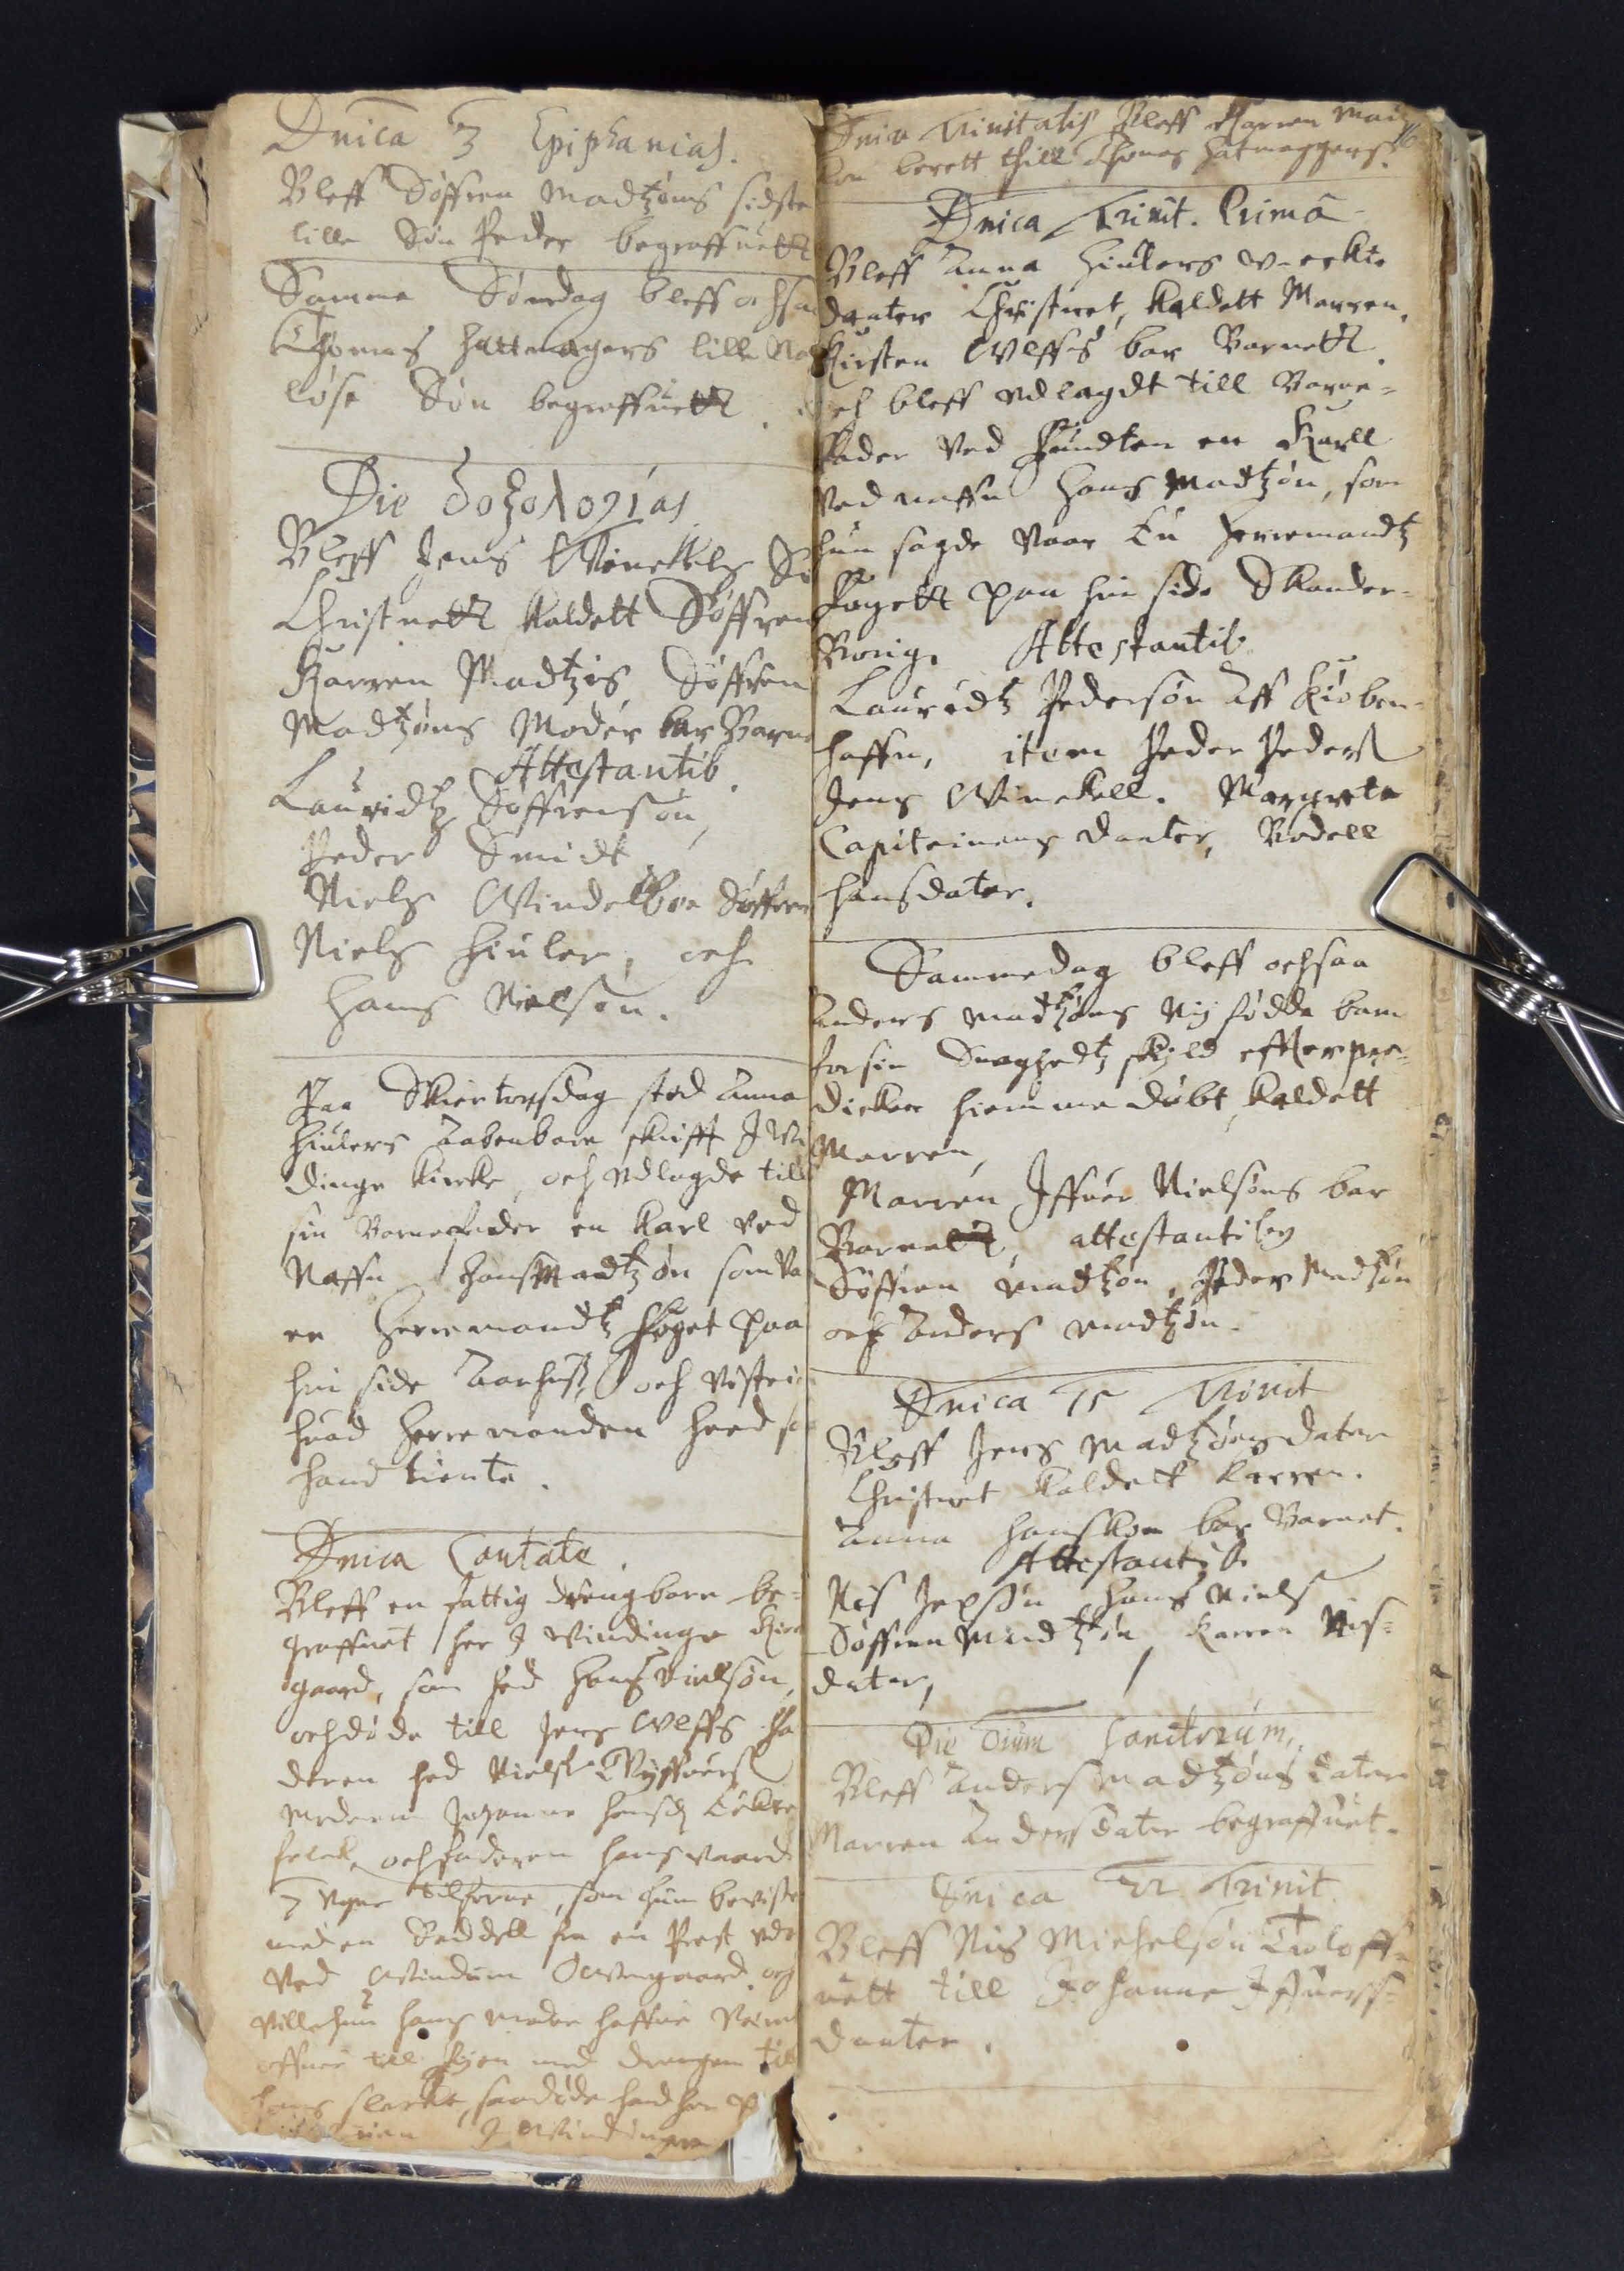Dnica 3 Epiphanias.
Bleff Søffren Madtzøns sidste
lille Søn Peder begraffuett
Samme Søndag bleff ochsaa
Thomas Hattemagers lille na 
løse Søn begraffuett
Die ##
Bleff Jens Winckels S 
Christnett kaldett Søffren
Karren Madtzis Søffren
Madtzøns Moder bar Barnet.
Attestantib
Lauridtz Søffrensøn
Peder Smidt
Niels Windelboe Søffren
Niels Hiuler, och
Hans Nielsøn
Paa Skiertorsdag stod Anna
Hiulers Aabenbare skrift I Wi  
dinge Kircke, och vdlagde till
sin Barnefader en karl ved
Naffn Hans Madtzøn som va¬
en Herremandtz Foget paa
hin side Aarhuss, och viste ic 
huad Herremanden heed
hand tiente
Dnica Cantate
Bleff en fattig drengebarn be¬
graffuet her I Windinge Kirc 
gaard, som hed Hans Nielsøn
och døde till Jens Vlffs. Fa
deren hed Niels Gijffuers
Moderen Johanne Hansd. Eckte
folck och faderen hans vaard
7 Vger tilforne## som hun beviste
med en Seddel fra en Præst
ved ##stind## ##stergaard och
ville hun hans moder haffue varet
pffuer til Fyen med drengen til
hans sleckt, saa døde hand for
## ## ## ##
16
Dnica Trinitatis. Bleff Karren Madtz
Kon berett thill Thomas Hatmaggers.
Dnica Trinit. Prima
Bleff Anna Hiulers v-eckte
daater Christnet, kaldett Marren
Kirsten Vlffs bar Barnett
 ch bleff udlagdt till Barne¬
fader ved Fundten en karll
ved naffn Hans Madtzøn, som
hun sagde vaar En Herremandtz
Fogett paa hin side Skander¬
Borig.
Attestantil
Lauridtz Pedersøn Aff Kiøben¬
haffn, item Peder Peders
Jens Winckell. Margreta
Capitainens daater. Bodell
Hansdaater.
Sammedag bleff ochsaa
Anders Madtzøns Ny fødde barn
for sin Suaghedtz skyld effter
dicken hiemme døbt, kaldett
Marren
Marren Iffuer Nielsøns bar
Barnett, Attestantiby
Søffren Madtzøn. Peder Madtzøn
och Anders Madtzøn
Dnica 15 Trinit
Bleff Jens Madtzøns dater
Christnet kaldett Karren.
Anna Hans Kone bar Barnet
Attestantib.
Nis Jepsøn Hans Niels
Søffren Madtzøn, Karren Nis¬
dater
Die Oium sanctorum.
Bleff Anders Madtzøns dater
Marren Andersdater begraffuet.
Dnica 22 Trinit.
Bleff Nis Michelsøn Troloff¬
uett till Johanne Iffuers¬
daater
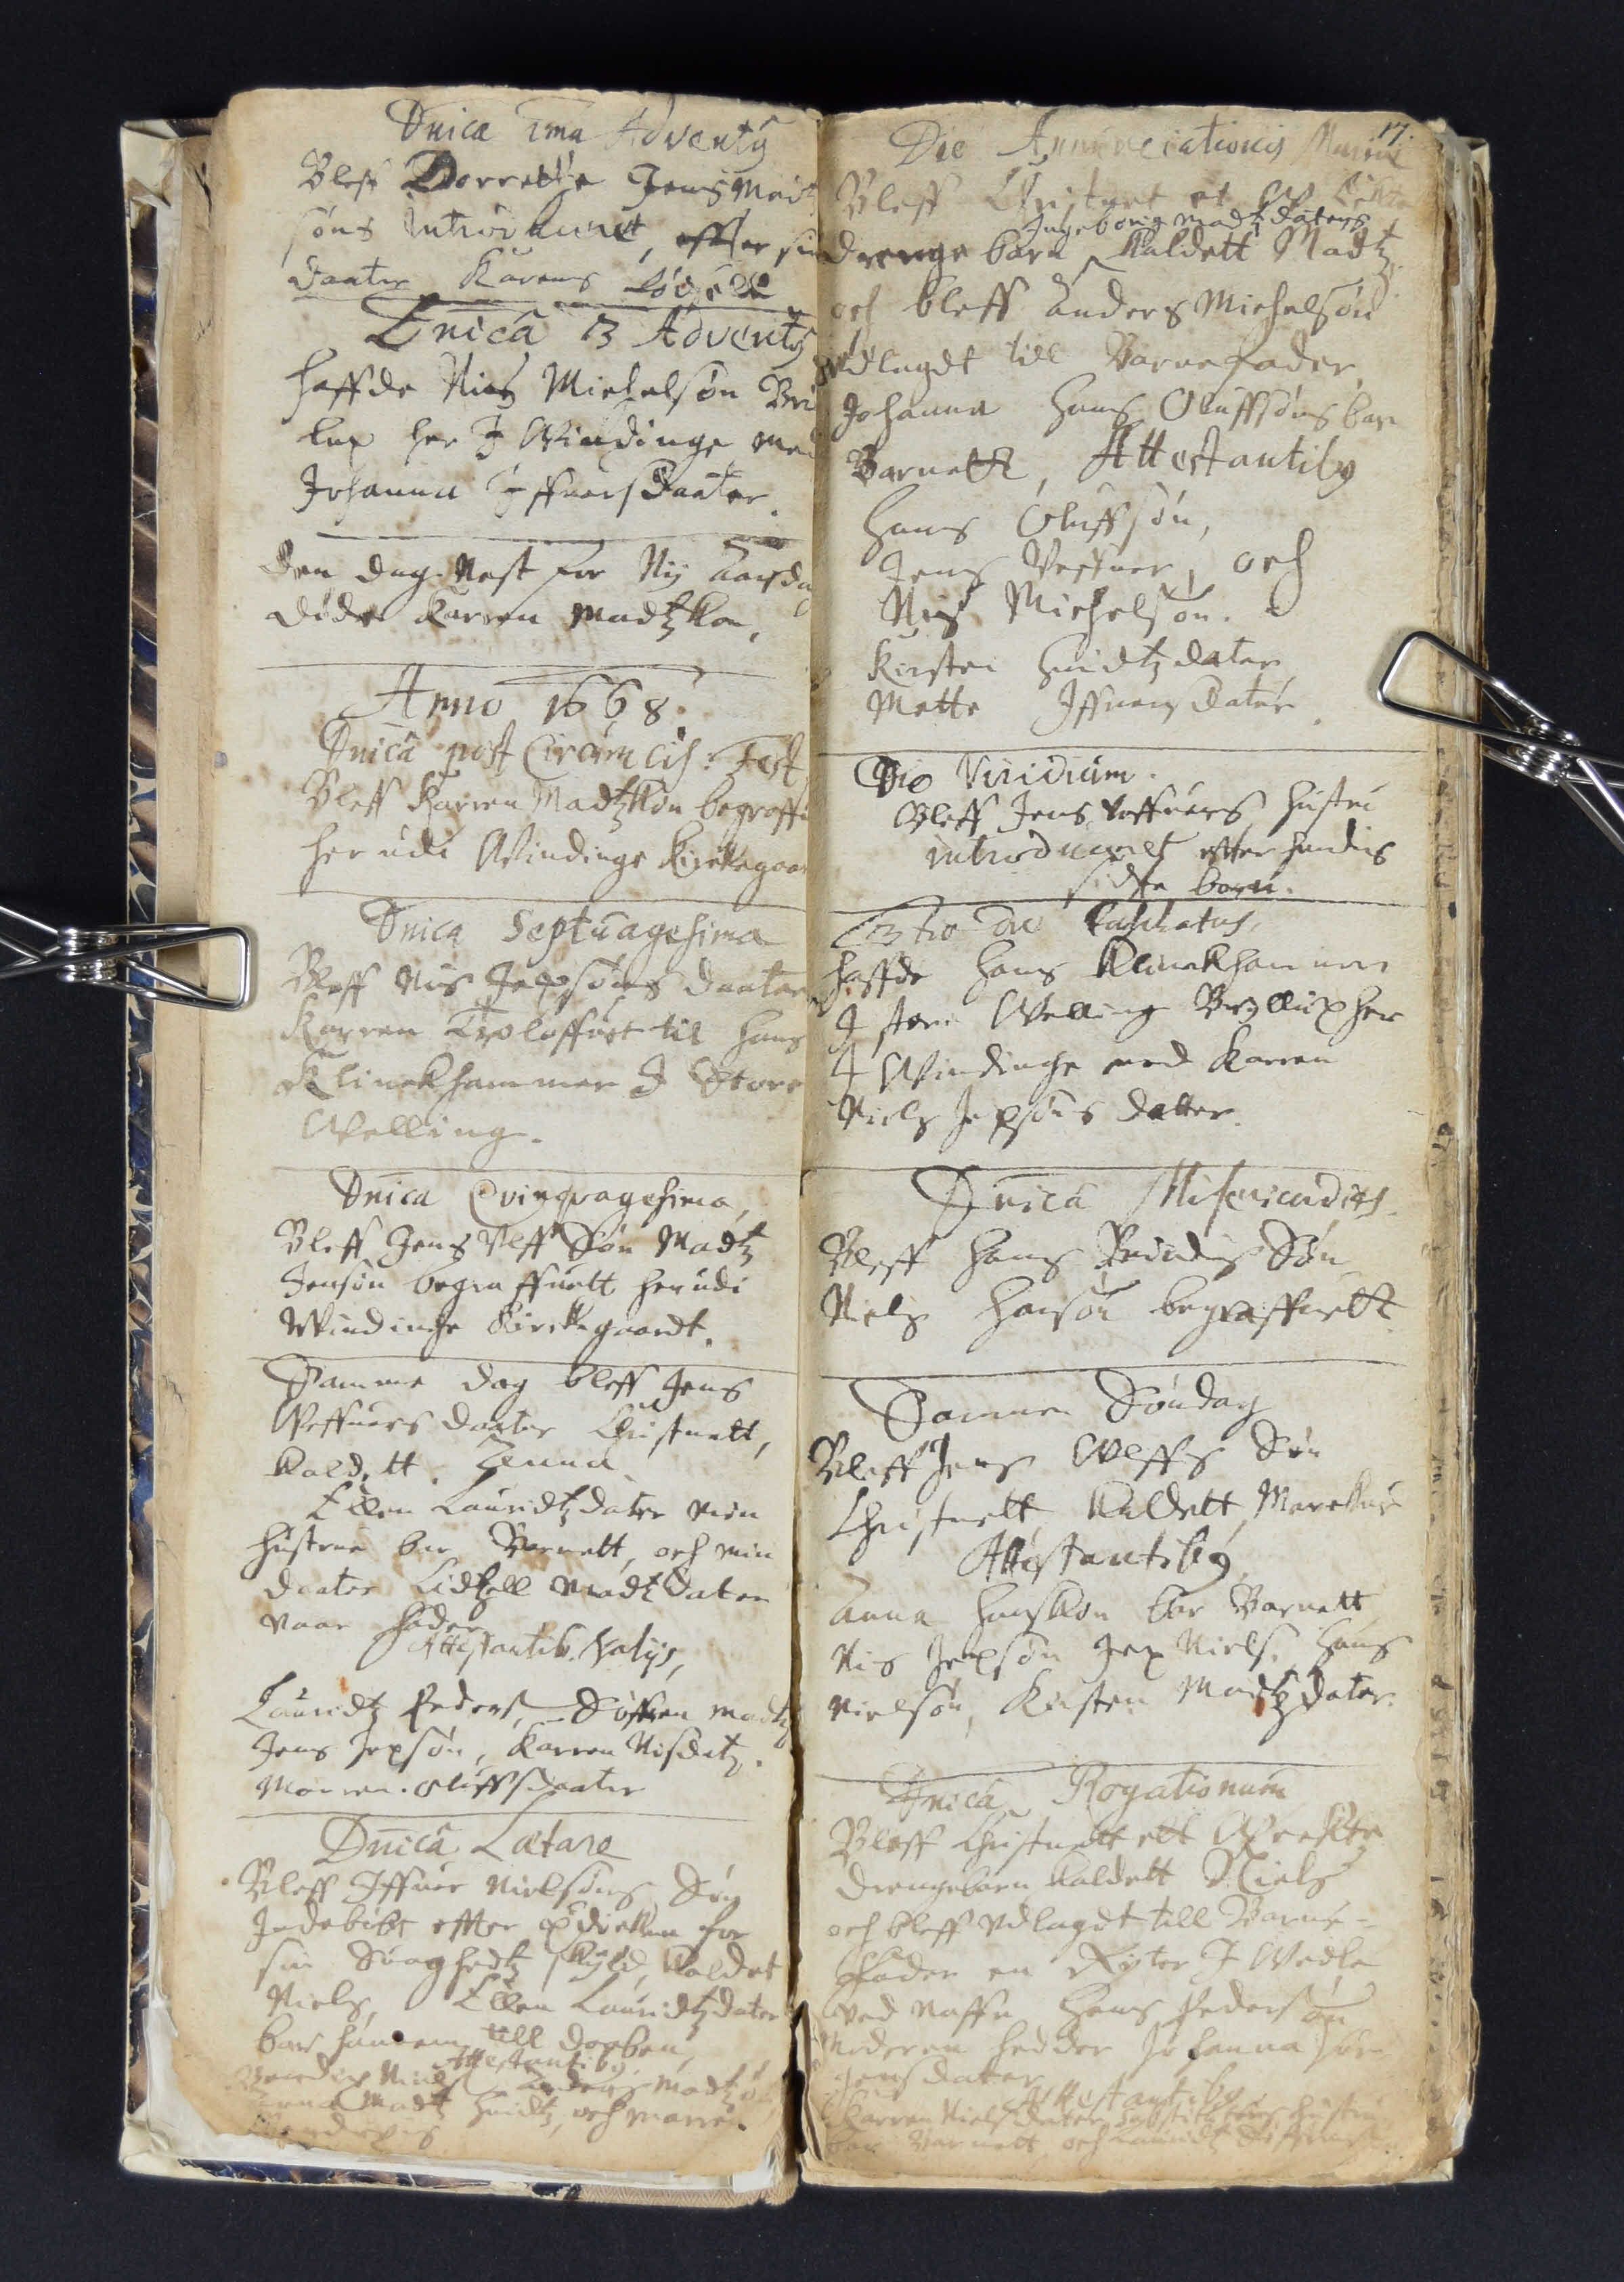Dnica 1 ma Adventus
Bleff Dorothe Jens Madtz
søns Introduceret, efter
Dnica 3 Adventus
daater Karrens
Haffde Niss Michelsøn
lup her I Windinge me 
Johanna Iffuersdaater
Den dag Nest for Ny Aarsdag
døde Karren Madtz kon
Anno 1668
Dnica post Circumcis: Fest
Bleff Karren Madtz Kon begraffu 
her udi Windinge Kirkegaar 
Dnica Septuagesima
Bleff Nis Jepsøns daater
Karren Troloffuet til Hans
Klinckhammer I Store
Welling
Dnica Quinquagesima
Bleff Jens Vlffs Søn Madtz
Jensøn begraffuett her udi
Windinge Kirkegaardt
Samme dag bleff Jens
Veffuers daater Christnet
kaldett. Anna
Ellen Lauridtzdater min
Hustrue bar Barnett, och min
daater Cidtzell Madtzdater
vaar fader. 
Attestantib. ##lys
Lauridtz Peders, Søffren Madtz
Jens Jepsøn. Karren Nisdat.
Marren Oluffsdaater
Dnica Latare
Bleff Iffuer Nielsøns Søn
Indebø## effter pdicken## for
sin suaghedtz skyld, kaldet
Niels, Ellen Lauridtzdater
bar hannem till daaben,
Attestantiby.
Bendix Niels Anders Madtzøn.
Anna Madtz Hvidtz, och Marren
Bendixis.
17.
Die Annunciationis Maria
Bleff Christnet et V Eckte
Ingeborig Madtzdatters
drenge barn kaldett Madtz
och bleff Anders Michelsøn
vdlagt till Barnefader
Johanna Hans Oluffsøns bar 
Barnett. Attestantiby
Hans Oluffsøn,
Jens Veffuer, och
Nis Michelsøn.
Kirsten Hvidtzdater
Mette Iffuersdater
Die Viridium
Bleff Jens Veffuers Hustru
introduceret efter hendis
sidste barn.
Festo do Paschatos
Haffde Hans Klinkhammer
I store Welling Bryllup her
I Windinge med Karren
Niels Jepsøns datter
Dnica Misericordias
Bleff Hans Bundes Søn
Niels Hansøn begraffuett
Samme Søndag
Bleff Jens Vlffs Søn
Christnett kaldett Marckus
Attestantiby
Anna Hans Kon bar Barnett
Nis Jepsøn. Jep Niels. Hans
Nielsøn. Kirsten Madtzdater.
Dnica Rogationum
Bleff Christens ett Veckte
Drengebarn kaldett Niels
och bleff vdlagt till Barne¬
Fader en Ryter I Wedle
ved naffn Hans Pedersøn
Moderen hedder Johanna Jør¬
gensdater.
Attestantiby
Karren Nielsdater Substituttens hustru
bar Barnet och Lauridtz Søffrens